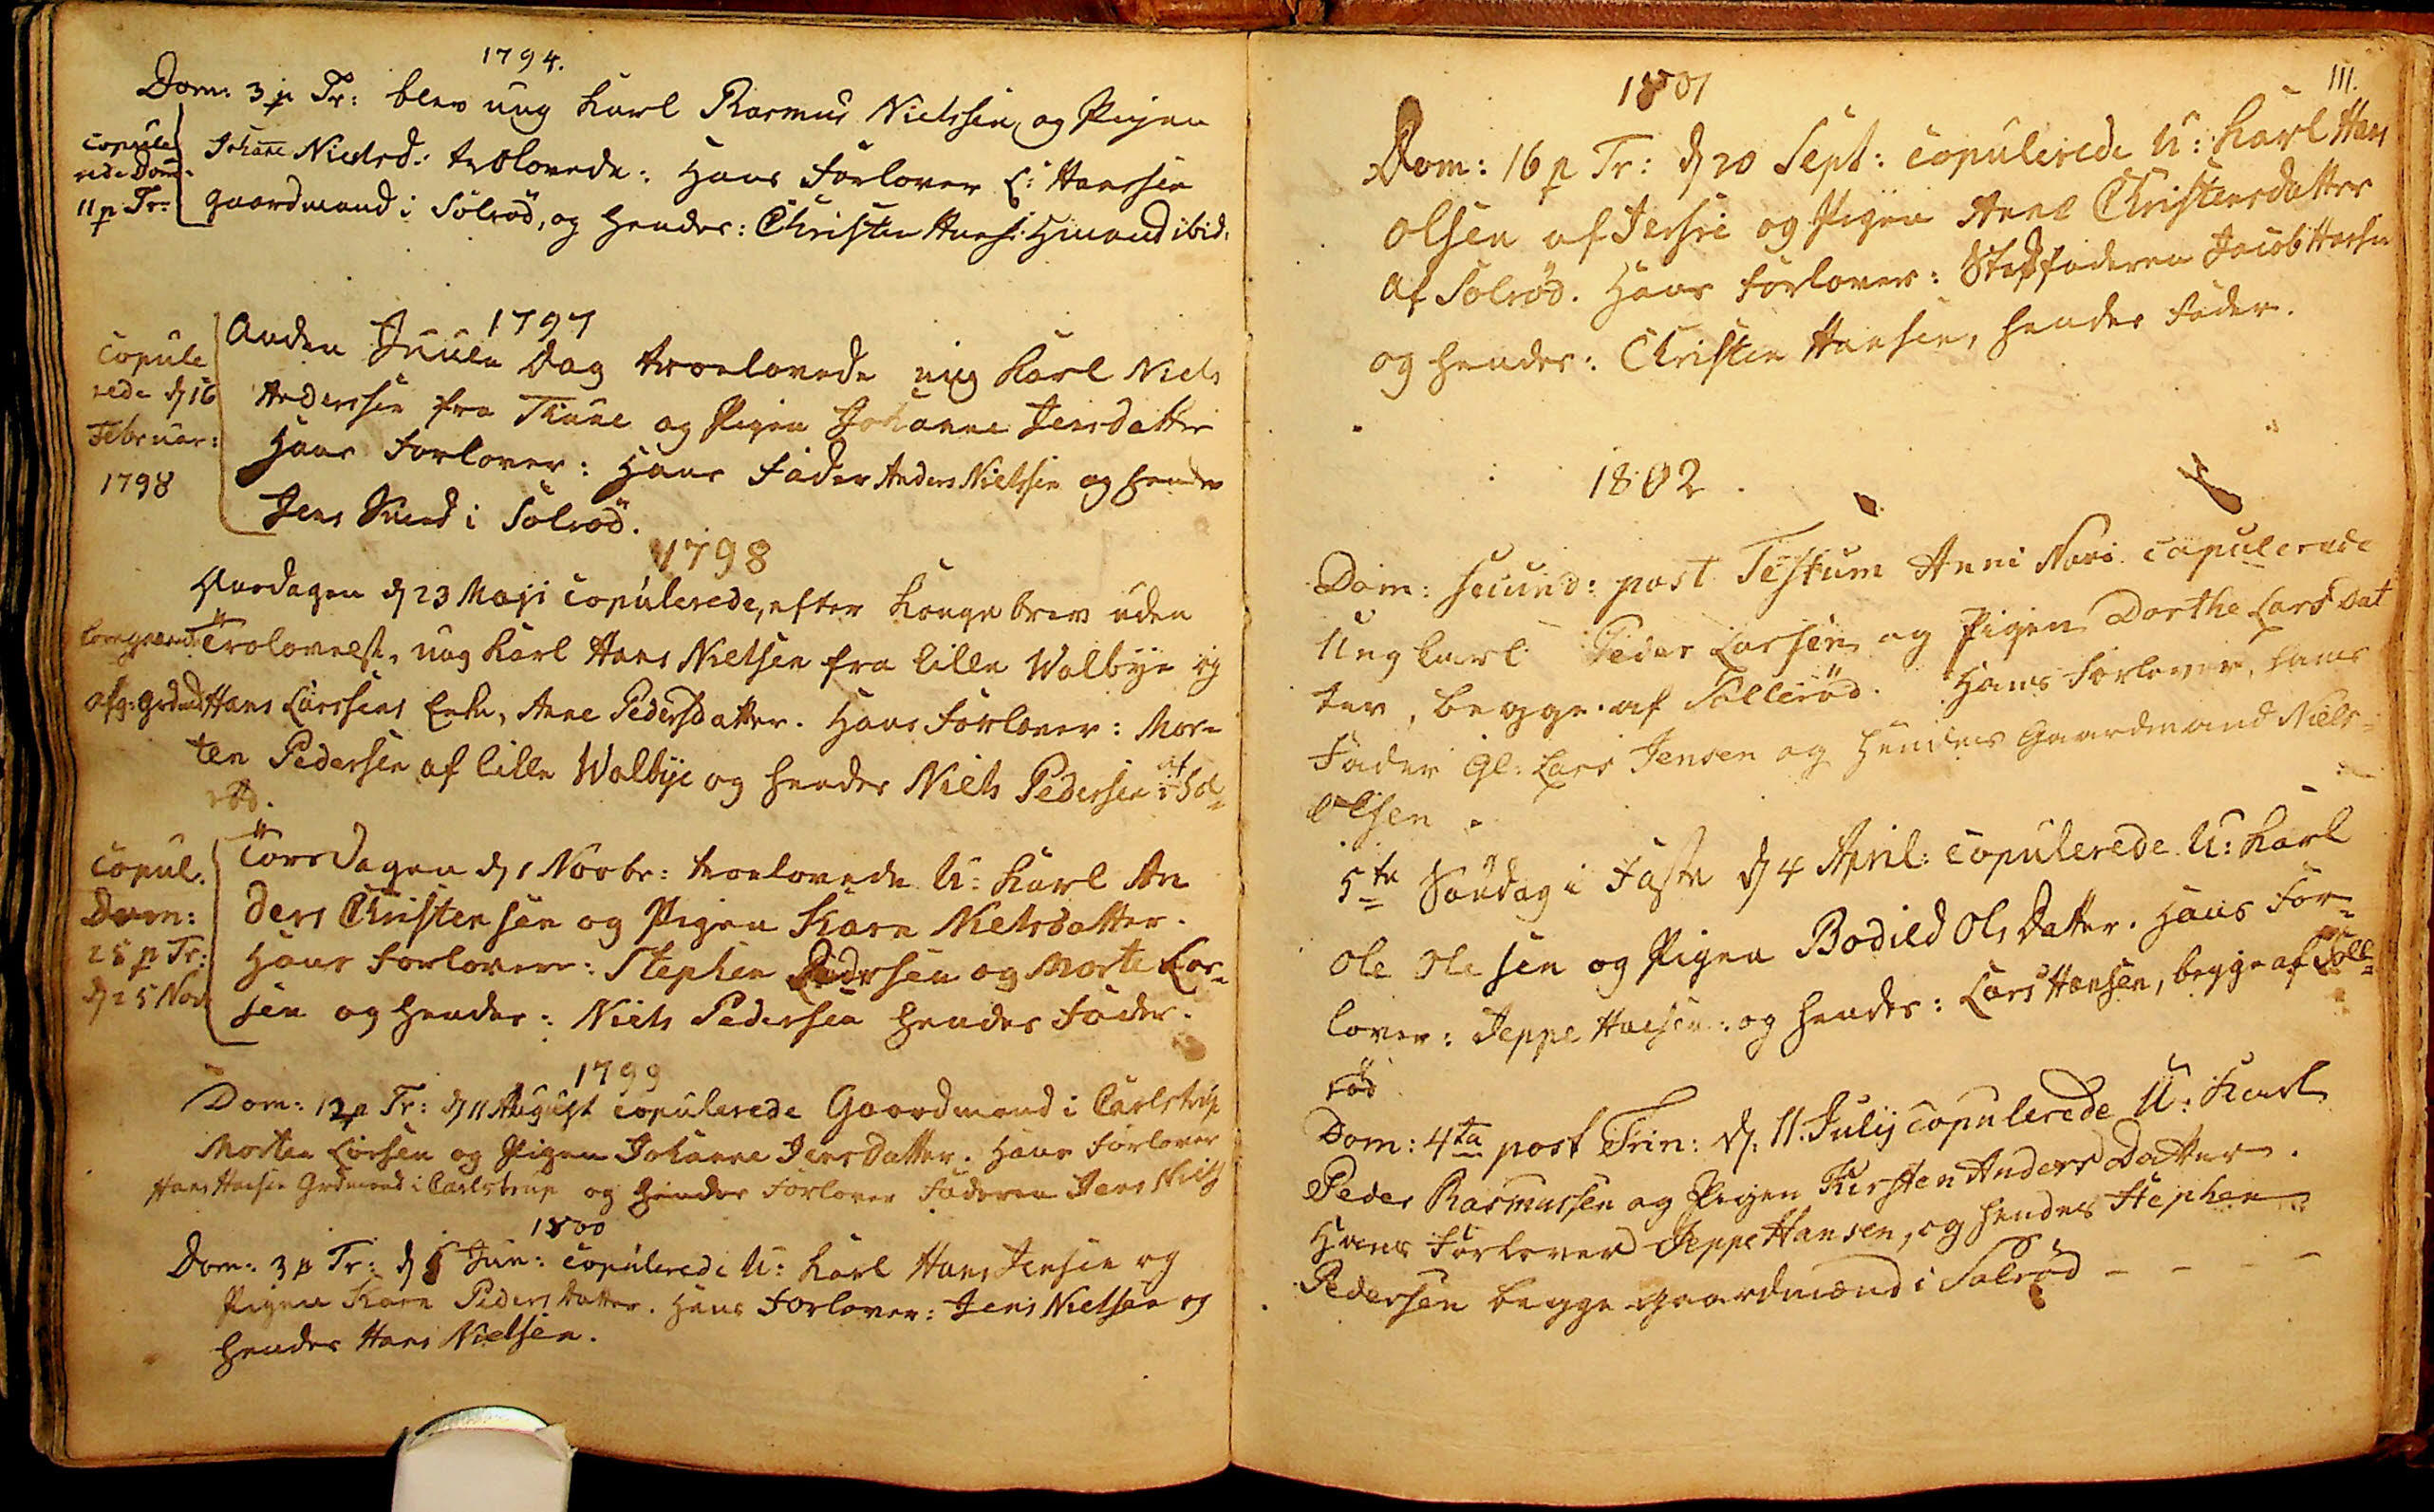1794.
Dom: 3 p Tr: blev ung Karl Rasmus Nielssen og Pigen
Johane Nielsd: trolovede: Hans Forlover L: Hanssen
Gaardmand i Solrød, og Hendes Christen Hans: Hmand ibid:
Copule
rede Dom:
11 p Tr:
1797 
Anden Juule Dag troelovede ung Karl Niels
Anderssen fra Thune og Pigen Johanne Jensdatter
Hans Forlover: Hans Fader Anders Nielssen og hendes
Jens Smed i Solrød.
Copule
rede d 16 
Februar:
1798
1798
Onsdagen d 23 Maji Copulerede efter Konge brev uden
Trolovelse, ung Karl Hans Nielsen fra lille Walbye og
Hans Larssens Enke, Anne Pedersdatter. Hans Forlover: Mor¬
ten Pedersen af lille Walbye og hendes Niels Pedersen af Sol¬
rød.
foregaaende
afg: Grdmd
Torsdagen d 1 Novbr: troelovede U:Karl An
ders Christensen og Pigen Karn Nielsdatter.
Hans Forlovere: Stephen Pedersen og Morten Lar¬
sen og hendes: Niels Pedersen hendes Fader.
Copul:
Dom:
25 p Tr:    
d 25 Nov:
1799
Dom: 12 p Tr: d 11 August Copulerede Gaardmand i Carlstrup
Morten Larsen og Pigen Johanne Jensdatter. Hans Forlover
Hans Hansen Grdmand i Carlstrup og hendes Forlover Faderen Jens Niels
1800
Dom: 3 p Tr: d 29 Jun: Copulerede U: Karl Hans Jensen og
Pigen Karn Peders Datter. Hans Forlover Jens Nielsen og
hendes Hans Nielsen.
111.
1801
Dom: 16 p Tr: d 20 Sept: Copulerede U:Karl Hans
Olsen af Jersie og Pigen Anne Christensdatter
af Solrød. Hans Forlover: Steffaderen Jacob Hansen
og hendes: Christen Hansen, hendes Fader.
1802
Dom: Secund: post Festum Anni Novi Copulerede
Ungkarl Peder Larsen og Pigen Dorthe LarsDat
ter, begge af Sollerød. Hans Forlover, hans
Fader Gl: Lars Jensen og Hendes Gaardmand Niels
Olsen.
5te Søndag i Faste d 4 April: Copulerede U:Karl
Ole Olesen og Pigen Bodil Ols Datter. Hans For¬
lover: Jeppe Hansen og hendes: Lars Hansen, begge af Solle¬
rød
Dom: 4ta post Trin: d: 11. Julij Copulerede U:Karl
Peder Rasmussen og Pigen Kirsten Anders Datter.
Hans Forlover Jeppe Hansen, og hendes Stephen
Pedersen begge Gaardmænd i Solrød
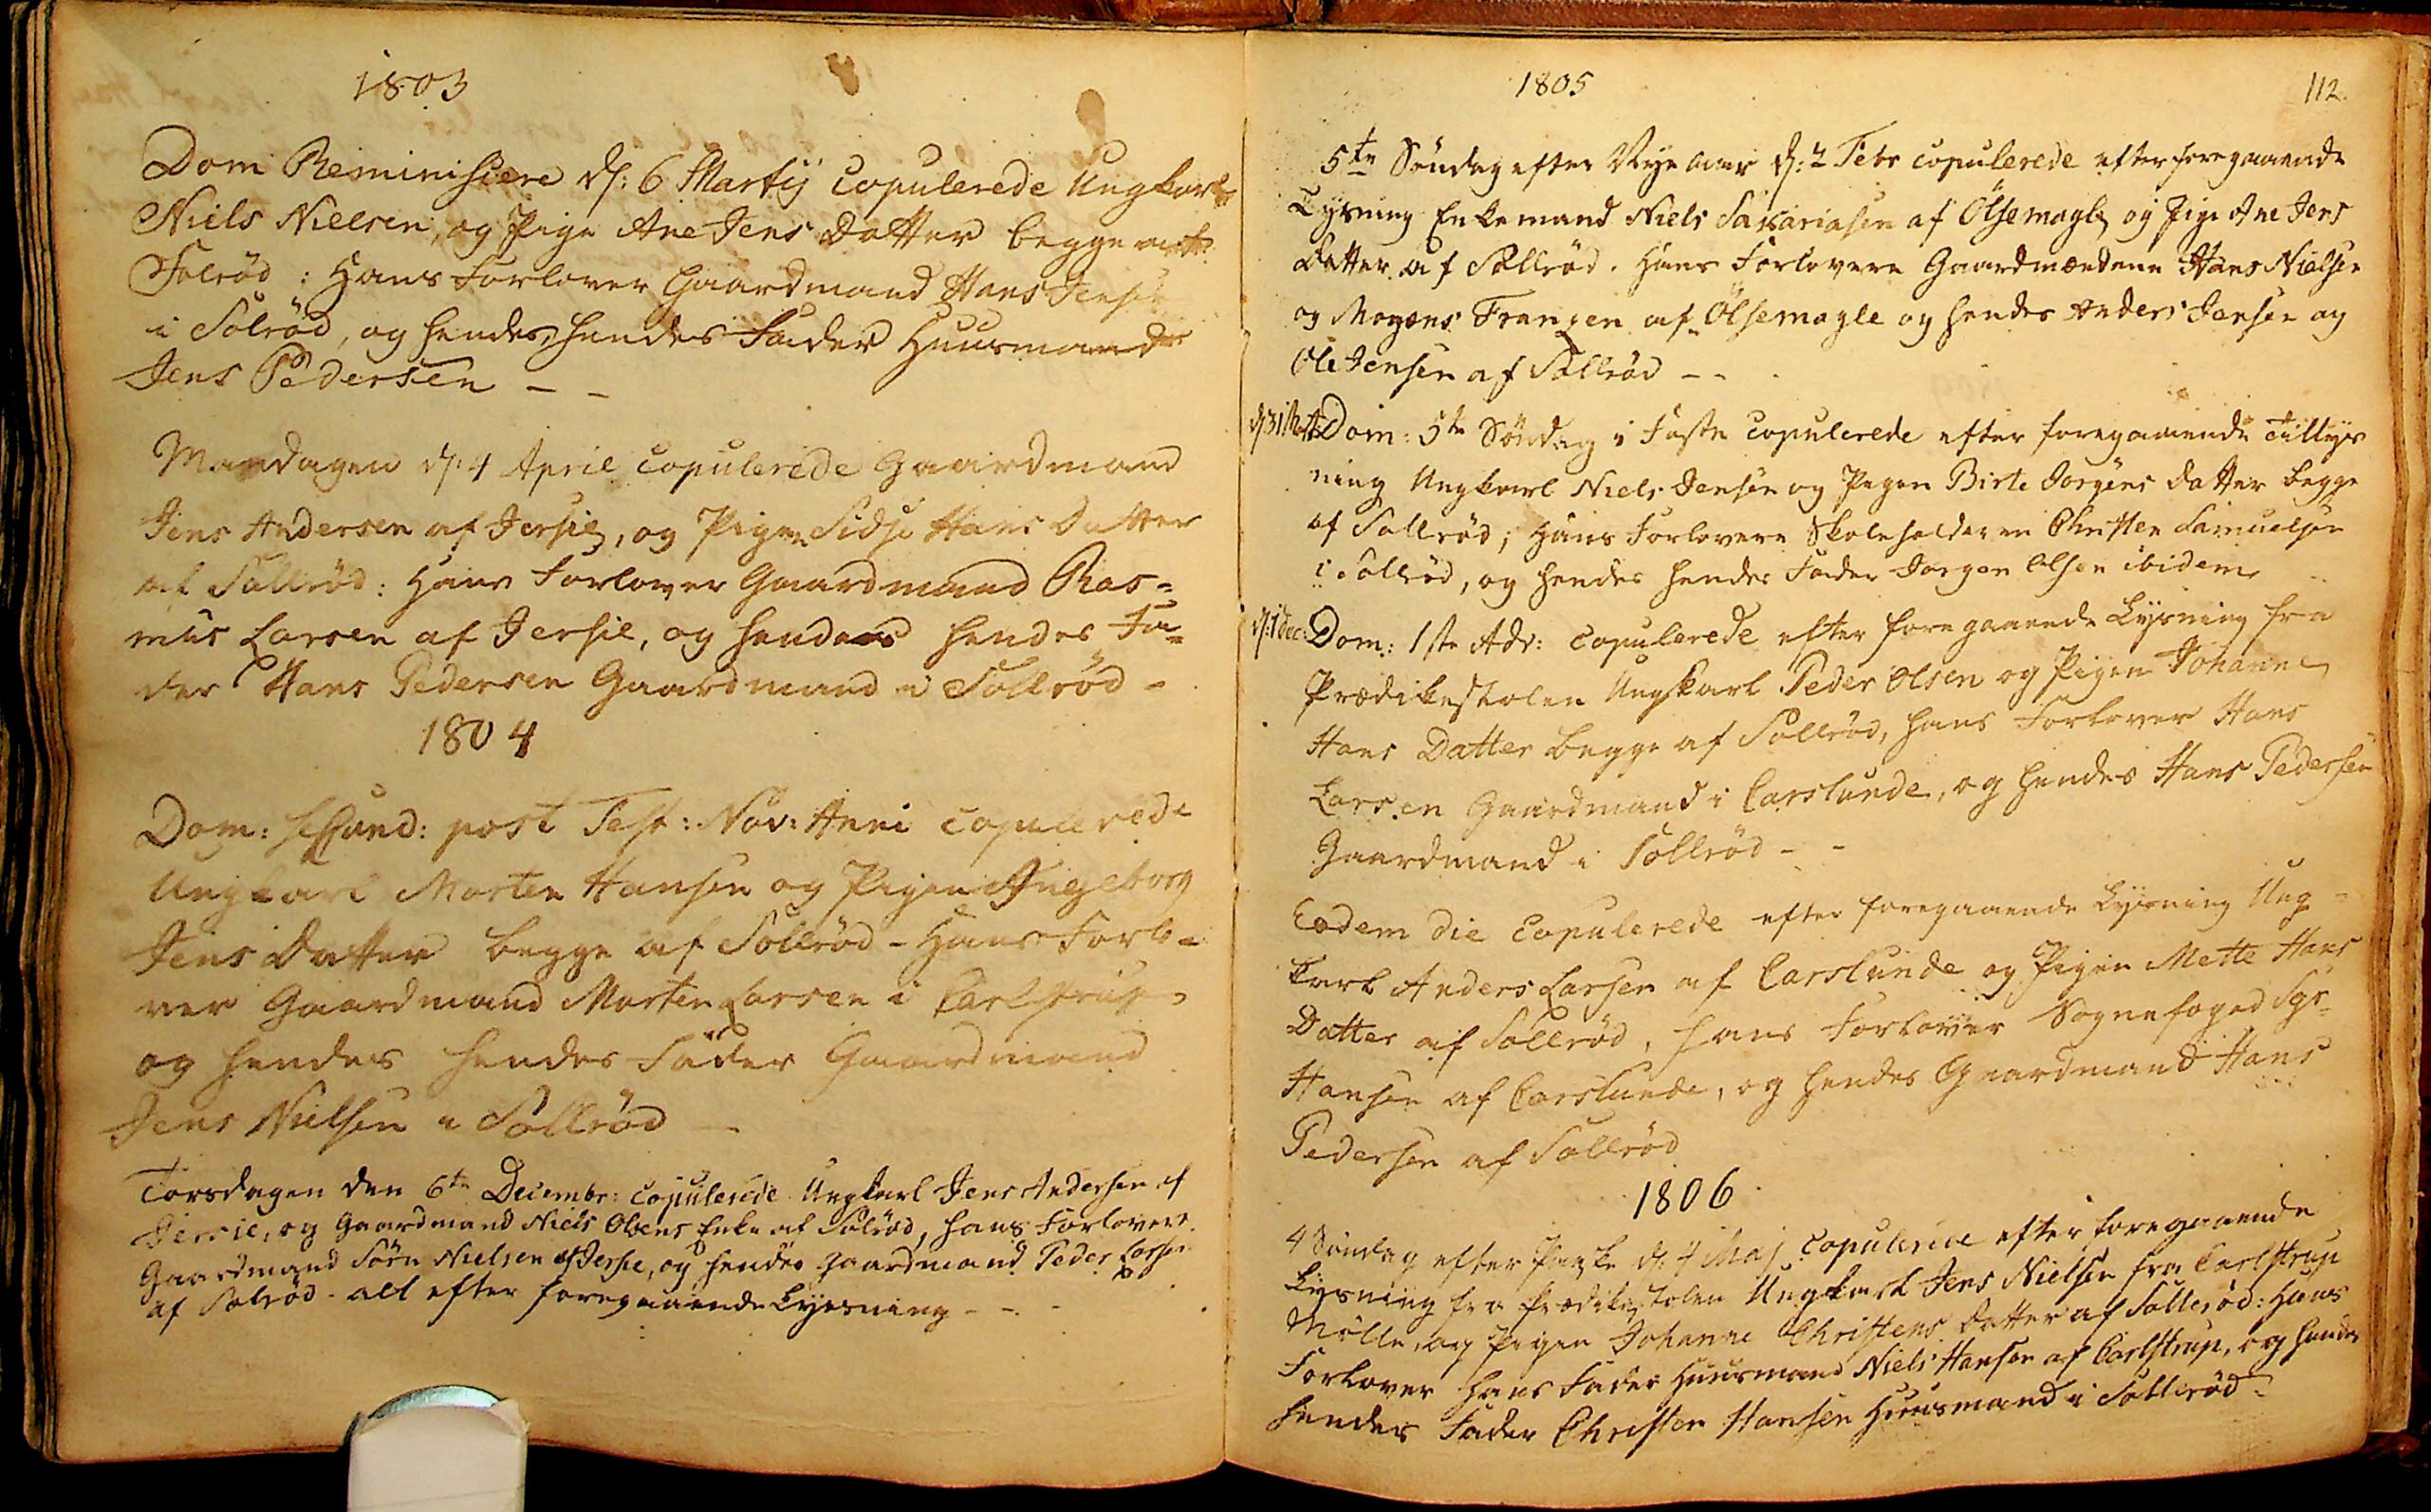1803
Dom Reminiscere d: 6 Martij Copulerede Ungkarl
Niels Nielsen, og Pige Ane Jens Datter begge af
Solrød: Hans Forlover Gaardmand Hans Jensen
i Solrød, og hendes, hendes Fader Huusmand
Jens Pedersen
Mandagen d: 4 April Copulerede Gaardmand
Jens Andersen af Jersie, og Pigen Sidse Hans Datter
af Sollrød: Hans Forlover Gaardmand Ras¬
mus Larsen af Jersie, og hendes hendes Fa¬
der Hans Pedersen Gaardmand i Sollrød -
1804
Dom: secund: post Fest: Nov: Anni Copulerede
Ungkarl Morten Hansen og Pigen Ingeborg
Jens Datter begge af Sollrød. Hans Forlo¬
ver Gaardmand Morten Larsen i Carlstrup
og hendes hendes Fader Gaardmand
Jens Nielsen i Sollrød
Torsdagen den 6te Decembr: Copulerede Ungkarl Jens Andersen af
Jersie, og Gaardmand Niels Olsens Enke af Solrød, hans Forlover
Gaardmand Sørn Nielsen af Jersie, og hendes Gaardmand Peder Larsen
af Solrød. alt efter foregaaende Lyesning
112
1805
5te Søndag efter Nye Aar d: 2 Febr Copulerede efter foregaaende
Lysning Enkemand Niels Sakariasen af Ølsemagle og Pige Ane Jens
Datter af Sollrød. Hans Forlovere Gaardmændene Hans Nielsen
og Mogens Franzen af Ølsemagle og hendes Anders Jensen og
Ole Jensen af Sollrød
d. 31 Marts Dom: 5te Søndag i Faste Copulerede efter foregaaende Tillys
ning Ungkarl Niels Jensen og Pigen Birte Jørgens Datter begge
af Sollrød; Hans Forlovere Skoleholderen Christen Samuelsen
i Sollrød, og hendes hendes Fader Jørgen Olsen ibidem
d: 1 dec: Dom: 1ste Adv: Copulerede efter foregaaende Lysning fra
Prædikestolen Ungkarl Peder Olsen og Pigen Johanne
Hans Datter begge af Sollrød, hans Forlover Hans
Larsen Gaardmand i Carlslunde. og hendes Hans Pedersen
Gaardmand i Sollrød
Eodem die Copulerede efter foregaaende Lysning Ung¬
karl Anders Larsen af Carlslunde og Pigen Mette Hans
Datter af Sollrød, hans Forlover Sognefoged Sgr
Hansen af Carlslunde, og hendes Gaardmand Hans
Pedersen af Sollrød
1806
4 Søndag efter Paaske d: 4 Maj Copulerede efter foregaaende
Lysning fra Prædikestolen Ungkarl Jens Nielsen fra Carlstrup
Mølle, og Pigen Johanne Christens Datter af Sollerød: Hans
Forlover hans Fader Huusmand Niels Hansen af Carlstrup, og hendes
hendes Fader Christen Hansen Huusmand i Sollerød.